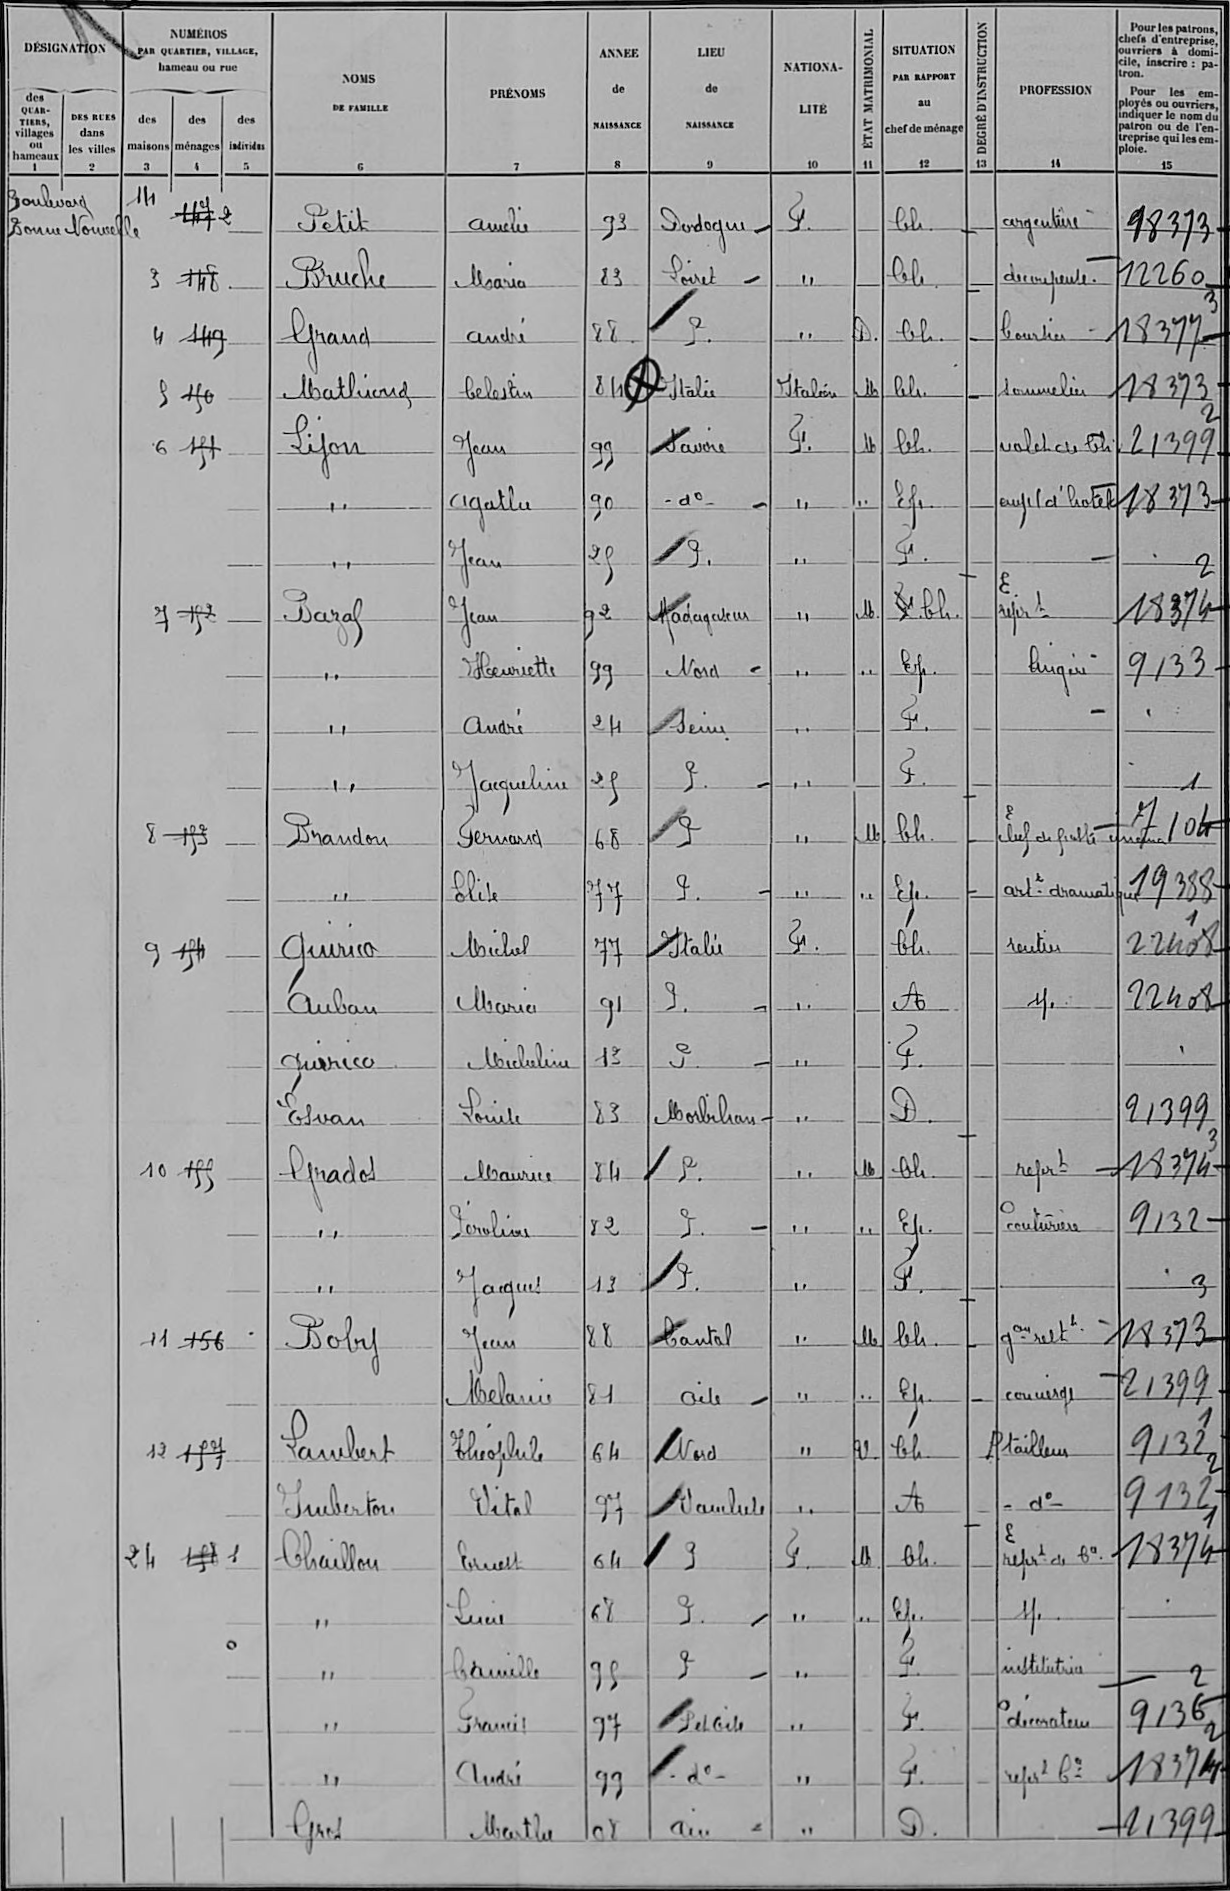Petit/amelie/93/Dordogne/F /¤/Ch /¤/argentière/18373
Bruche/Maria/83/Loiret/''/¤/Ch /¤/decoupeuse/12260
Grand/andré/88/P /''/D /Ch /¤/boursier/18377
Mathioud/Celestin/84/Italie/Italien/M/Ch /¤/sommelier/18373
Lijon/Jean/99/Savoie/F /M/Ch /¤/valet de ch/21399
''/Agathe/90/-d°/''/''/Ep /¤/empl d'hotel/18373
''/Jean/25/P /''/¤/F /¤/¤/2
Bazal/Jean/92/Madagascar/''/M/ch /¤/refer/18374
''/Henriette/99/Nord/''/''/Ep /¤/lingère/9133
''/André/24/Seine/''/¤/F /¤/¤/¤
''/Jacqueline/25/P /''/¤/F /¤/¤/1
Brandon/Fernand/68/P/''/M/Ch/¤/chef de fiacre unena/?7104
''/Elise/77/P /''/''/Ep /¤/art dramatique/19388
Guirico/Michel/77/Italie/F /¤/Ch /¤/rentier/22408
Auban/Marie/91/P/''/¤/A/¤/sp /22408
Guirico/Micheline/13/P/"/¤/F/¤/¤/¤
Esvan/Louise/83/Morbihan/''/¤/D/¤/¤/21399
Grados/Maurice/84/P /''/M/Ch /¤/refer t/18374
''/Péroline/82/P /''/''/Ep /¤/Couturière/9132
''/Jacques/13/P /''/¤/F /¤/¤/3
Boby/Jean/88/Cantal/''/M/Ch /¤/gon relt/18373
¤/Melanie/81/Oise/''/''/Ep /¤/concierge/21399
Lambert/Théophile/64/Nord/''/V /Ch /¤/P tailleur/9132
Iruberton/Vital/97/Vaucluse/''/¤/A/¤/d°/9132
Chaillon/Ernest/64/P/F /M/Ch /¤/repr de C/18374
''/Lucie/68/P /''/''/Ep /¤/sp/¤
''/Camille/95/P/''/¤/F /¤/institutrice/¤
''/Francis/97/S et Oise/''/¤/F /¤/décorateur/9136
''/André/99/d°/''/¤/F /¤/repr C/18374
Gros/Marthe/08/Ain/''/¤/D /¤/¤/21399
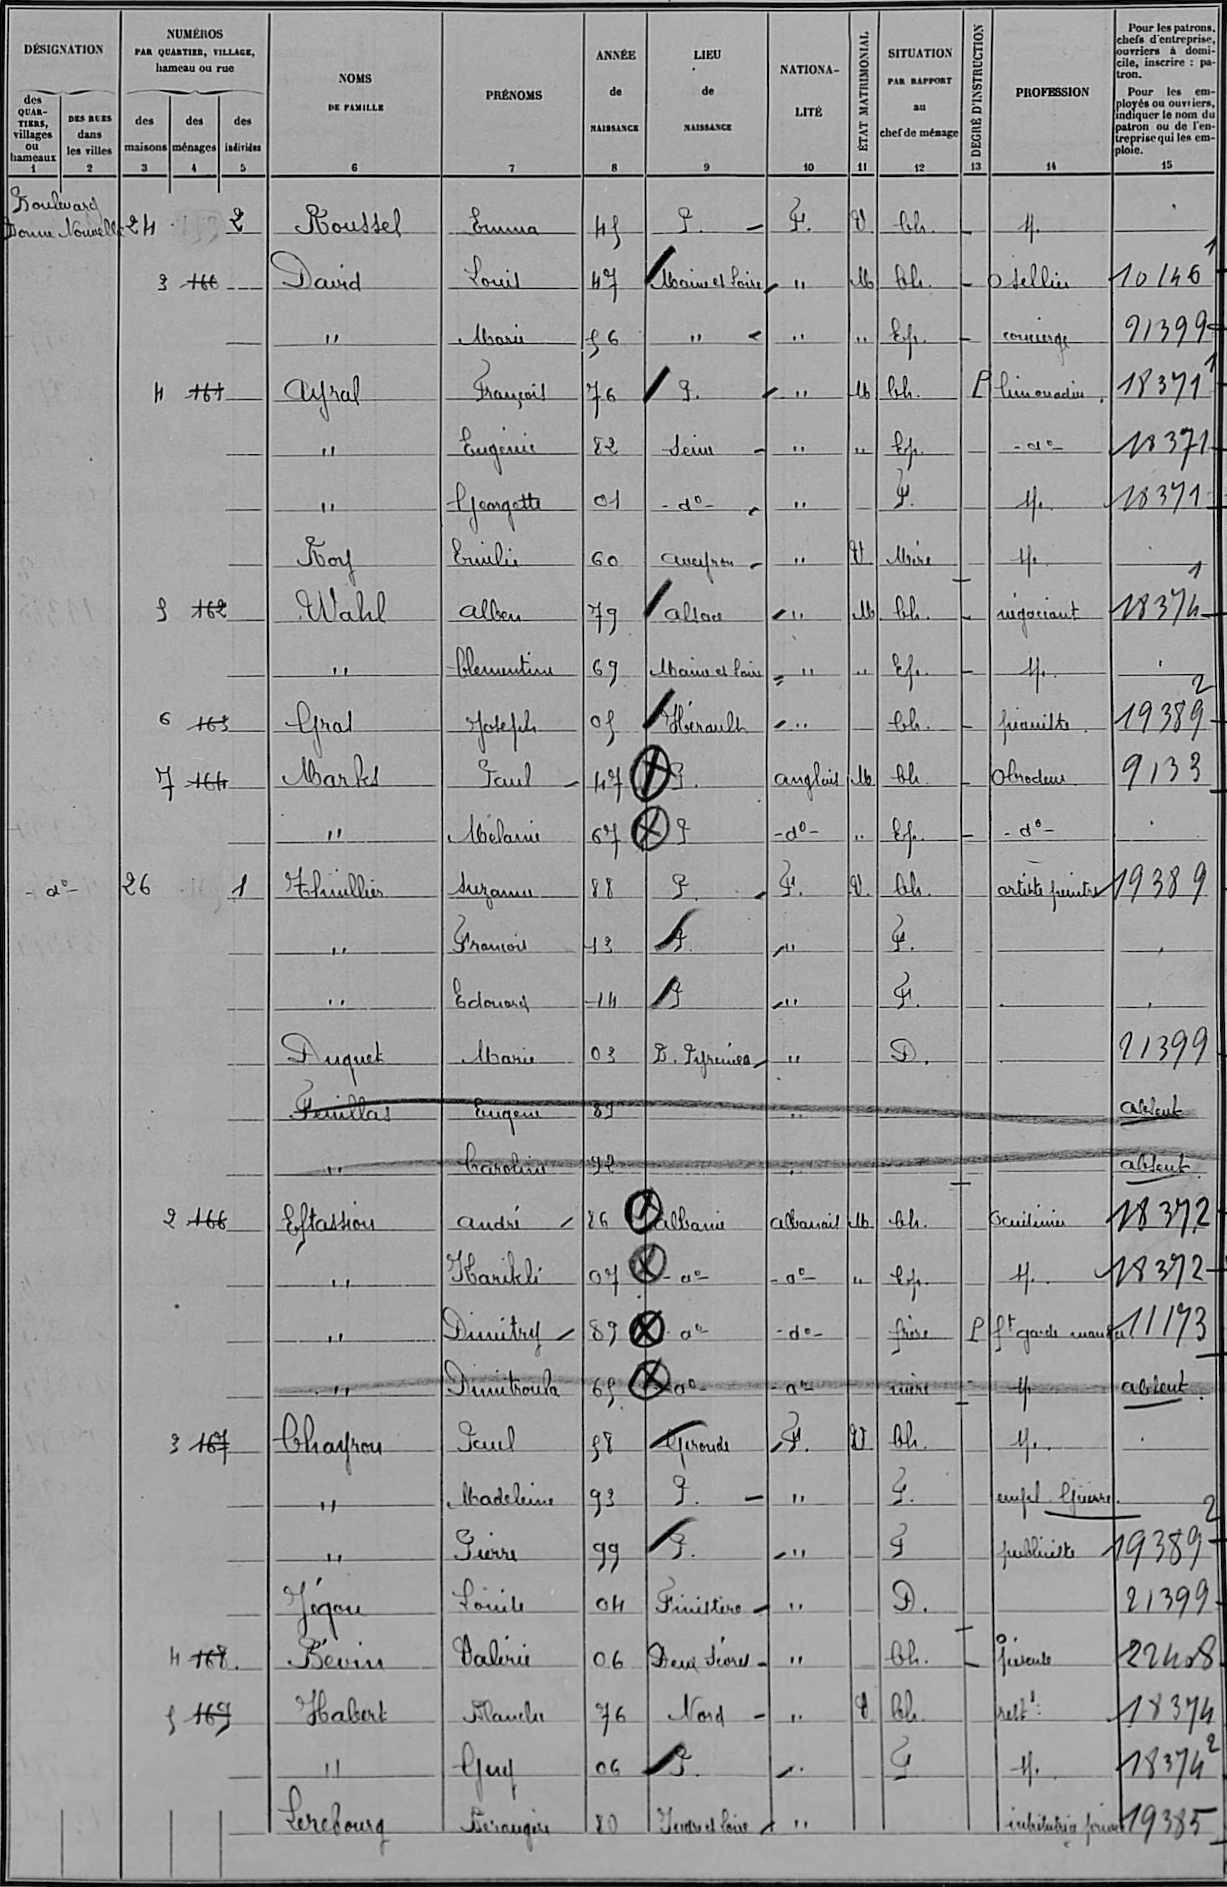Roussel/Emma/45/P /F /V/Ch /¤/sp /¤
David/Louis/47/Maine et Loire/"/M/Ch /¤/O Sellier/10146
""/Marie/56/"/"/"/Ep /¤/conceirge/91399
Ayral/François/76/P /"/M/Ch /¤/P limonadier/18371
""/Eugénie/82/Seine/"/"/Ep /¤/d°/18371
""/Georgette/01/d°/"/¤/F /¤/sp /18371
Roy/Emilie/60/Aveyron/"/V/Mère/¤/sp /¤
Wahl/Alben/79/Alsace/"/M /Ch /¤/négociant/18374
"/Clementine/69/Maine et Loire/"/"/Ep /¤/sp /¤
Gras/Joseph/05/Hérault/"/¤/Ch /¤/pianiste/19389
Marks/Paul/47/P /anglais/M/Ch /¤/o brodeur/9133
""/Mélanie/67/P/d°/"/Ep /¤/d°/¤
Thuillier/Suzanne/88/P /F /V /Ch /¤/artiste peintre/19389
""/François/13/P /"/¤/F /¤/¤/¤
""/Edouard/14/P/"/¤/F /¤/¤/¤
Duquet/Marie/03/B Pyrénées/"/¤/D /¤/¤/21399
Feuillas/Eugénie/89/¤/¤/¤/¤/¤/¤/absent
""/Caroline/92/¤/¤/¤/¤/¤/¤/absent
Eftassion/André/86/Albanie/Albanais/M /Ch /¤/O cuisinier/18372
""/Harikli/07/d°/d°/"/Ep /¤/Sp /18372
""/Dimitry/89/d°/d°/¤/frère/¤/P ft garde manger/11173
""/Dimitroula/65/d°/d°/¤/mère/¤/sp/absent
Chayron/Paul/58/Gironde/F /V/Ch /¤/Sp /¤
"/Madeleine/93/P /"/¤/F /¤/empl Guerre/¤
""/Pierre/99/P /"/¤/F/¤/publiciste/19389
Jégou/Louise/04/Finistère/"/¤/D /¤/¤/21399
Bévin/Valérie/06/Deux Sèvres/"/¤/Ch /¤/serveuse?o/22408
Habert/Blanche/76/Nord/"/V/Ch /¤/restt/18374
""/Guy/06/P /¤/¤/F/¤/sp /18374
Lerebourg/Bérangère/80/Indre et Loire/"/¤/¤/¤/institutrice privée/19385